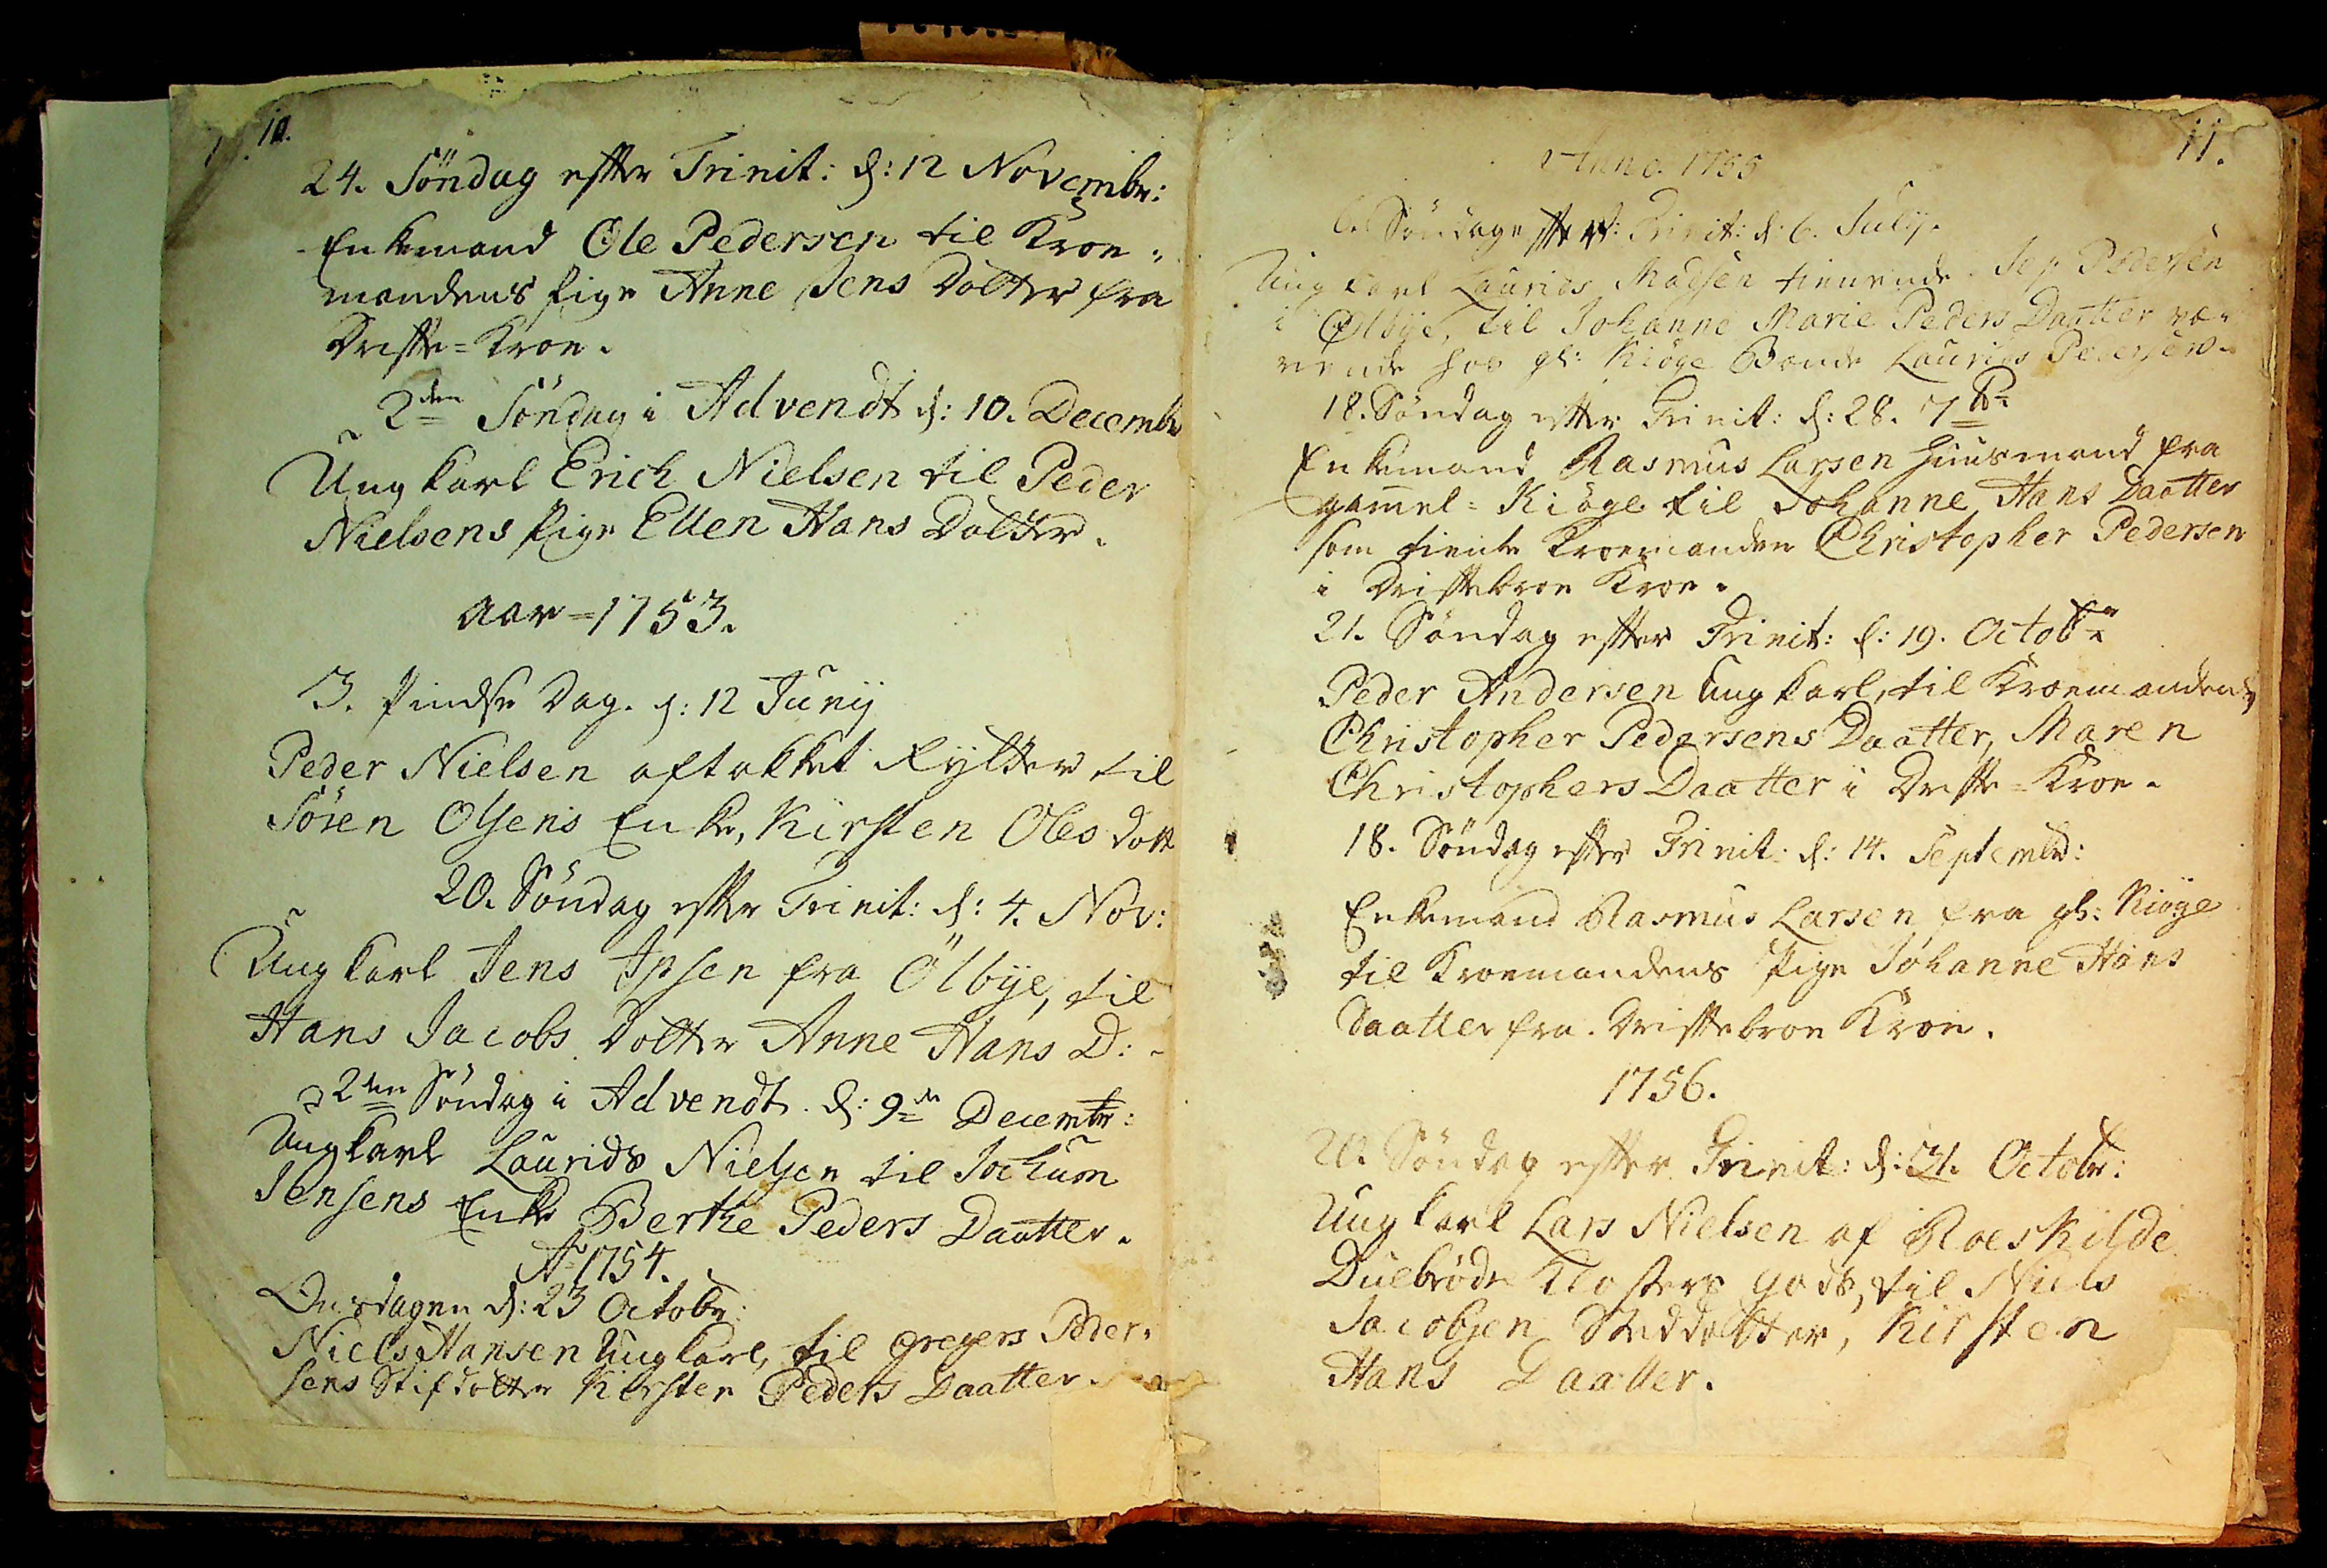10.
24. Søndag efter Trinit: d: 12 Novembr:
Enkemand Ole Pedersen til Kroe¬
mandens Pige Anne Jens Datter fra 
Drifte=Kroe.
2den Søndag i Advendt d: 10. Decembr
Ungkarl Erich Nielsen til Peder
Nielsens Pige Ellen Hans Datter.
Aar = 1753.
3. Pindse Dag. d: 12 Junij
Peder Nielsen aftakket Rytter til 
Søren Olsens Enke, Kirsten Olesdatt
20. Søndag efter Trinit: d: 4. Nov:
Ungkarl Jens Ipsen fra Ølbye, til
Hans Jacobs Datter Anne Hans D:
2den Søndag i Advendt. d: 9de Decembr:
Ungkarl Laurids Nielsen til Jochum
Jensens Enke Berthe Peders Daatter.
Ao 1754.
Onsdagen d: 23 Octobr:
Niels Hansen Ungkarl, til Gregers Peder¬
sens Stifdatter Kirsten Peders Daatter.
11.
Anno 1755
6. Søndag efter D: Trinit: d: 6. Julij.
Ungkarl Laurids Madsen tienende Jep Pedersen
i Ølbye, til Johanne Marie Peders Daatter væ¬
rende hos gl: Kiøge Bonde Laurids Pedersen.
18. Søndag efter Trinit: d: 28. 7br
Enkemand Rasmus Larsen Huusmand fra
Gammel = Kiøge til Johanne Hans Daatter
som tiente Kroemanden Christopher Pedersen
i Driftebroe Kroe.
21. Søndag efter Trinit: d: 19. Octobr.
Peder Andersen Ungkarl, til Kroemandens
Christopher Pedersens Daatter, Maren
Christophers Daatter i Drifte = Kroe.
18. Søndag efter Trinit: d: 14. Septembr:
Enkemand Rasmus Larsen fra gl: Kiøge
til Kroemandens Pige Johanne Hans
Daatter fra Driftebroe Kroe
1756.
20. Søndag efter Trinit: d: 31. Octobr:
Ungkarl Lars Nielsen af Roeskilde
Duebrødr Klosters Gods, til Niels
Jacobsen Steddatter, Kirsten
Hans Daatter. 
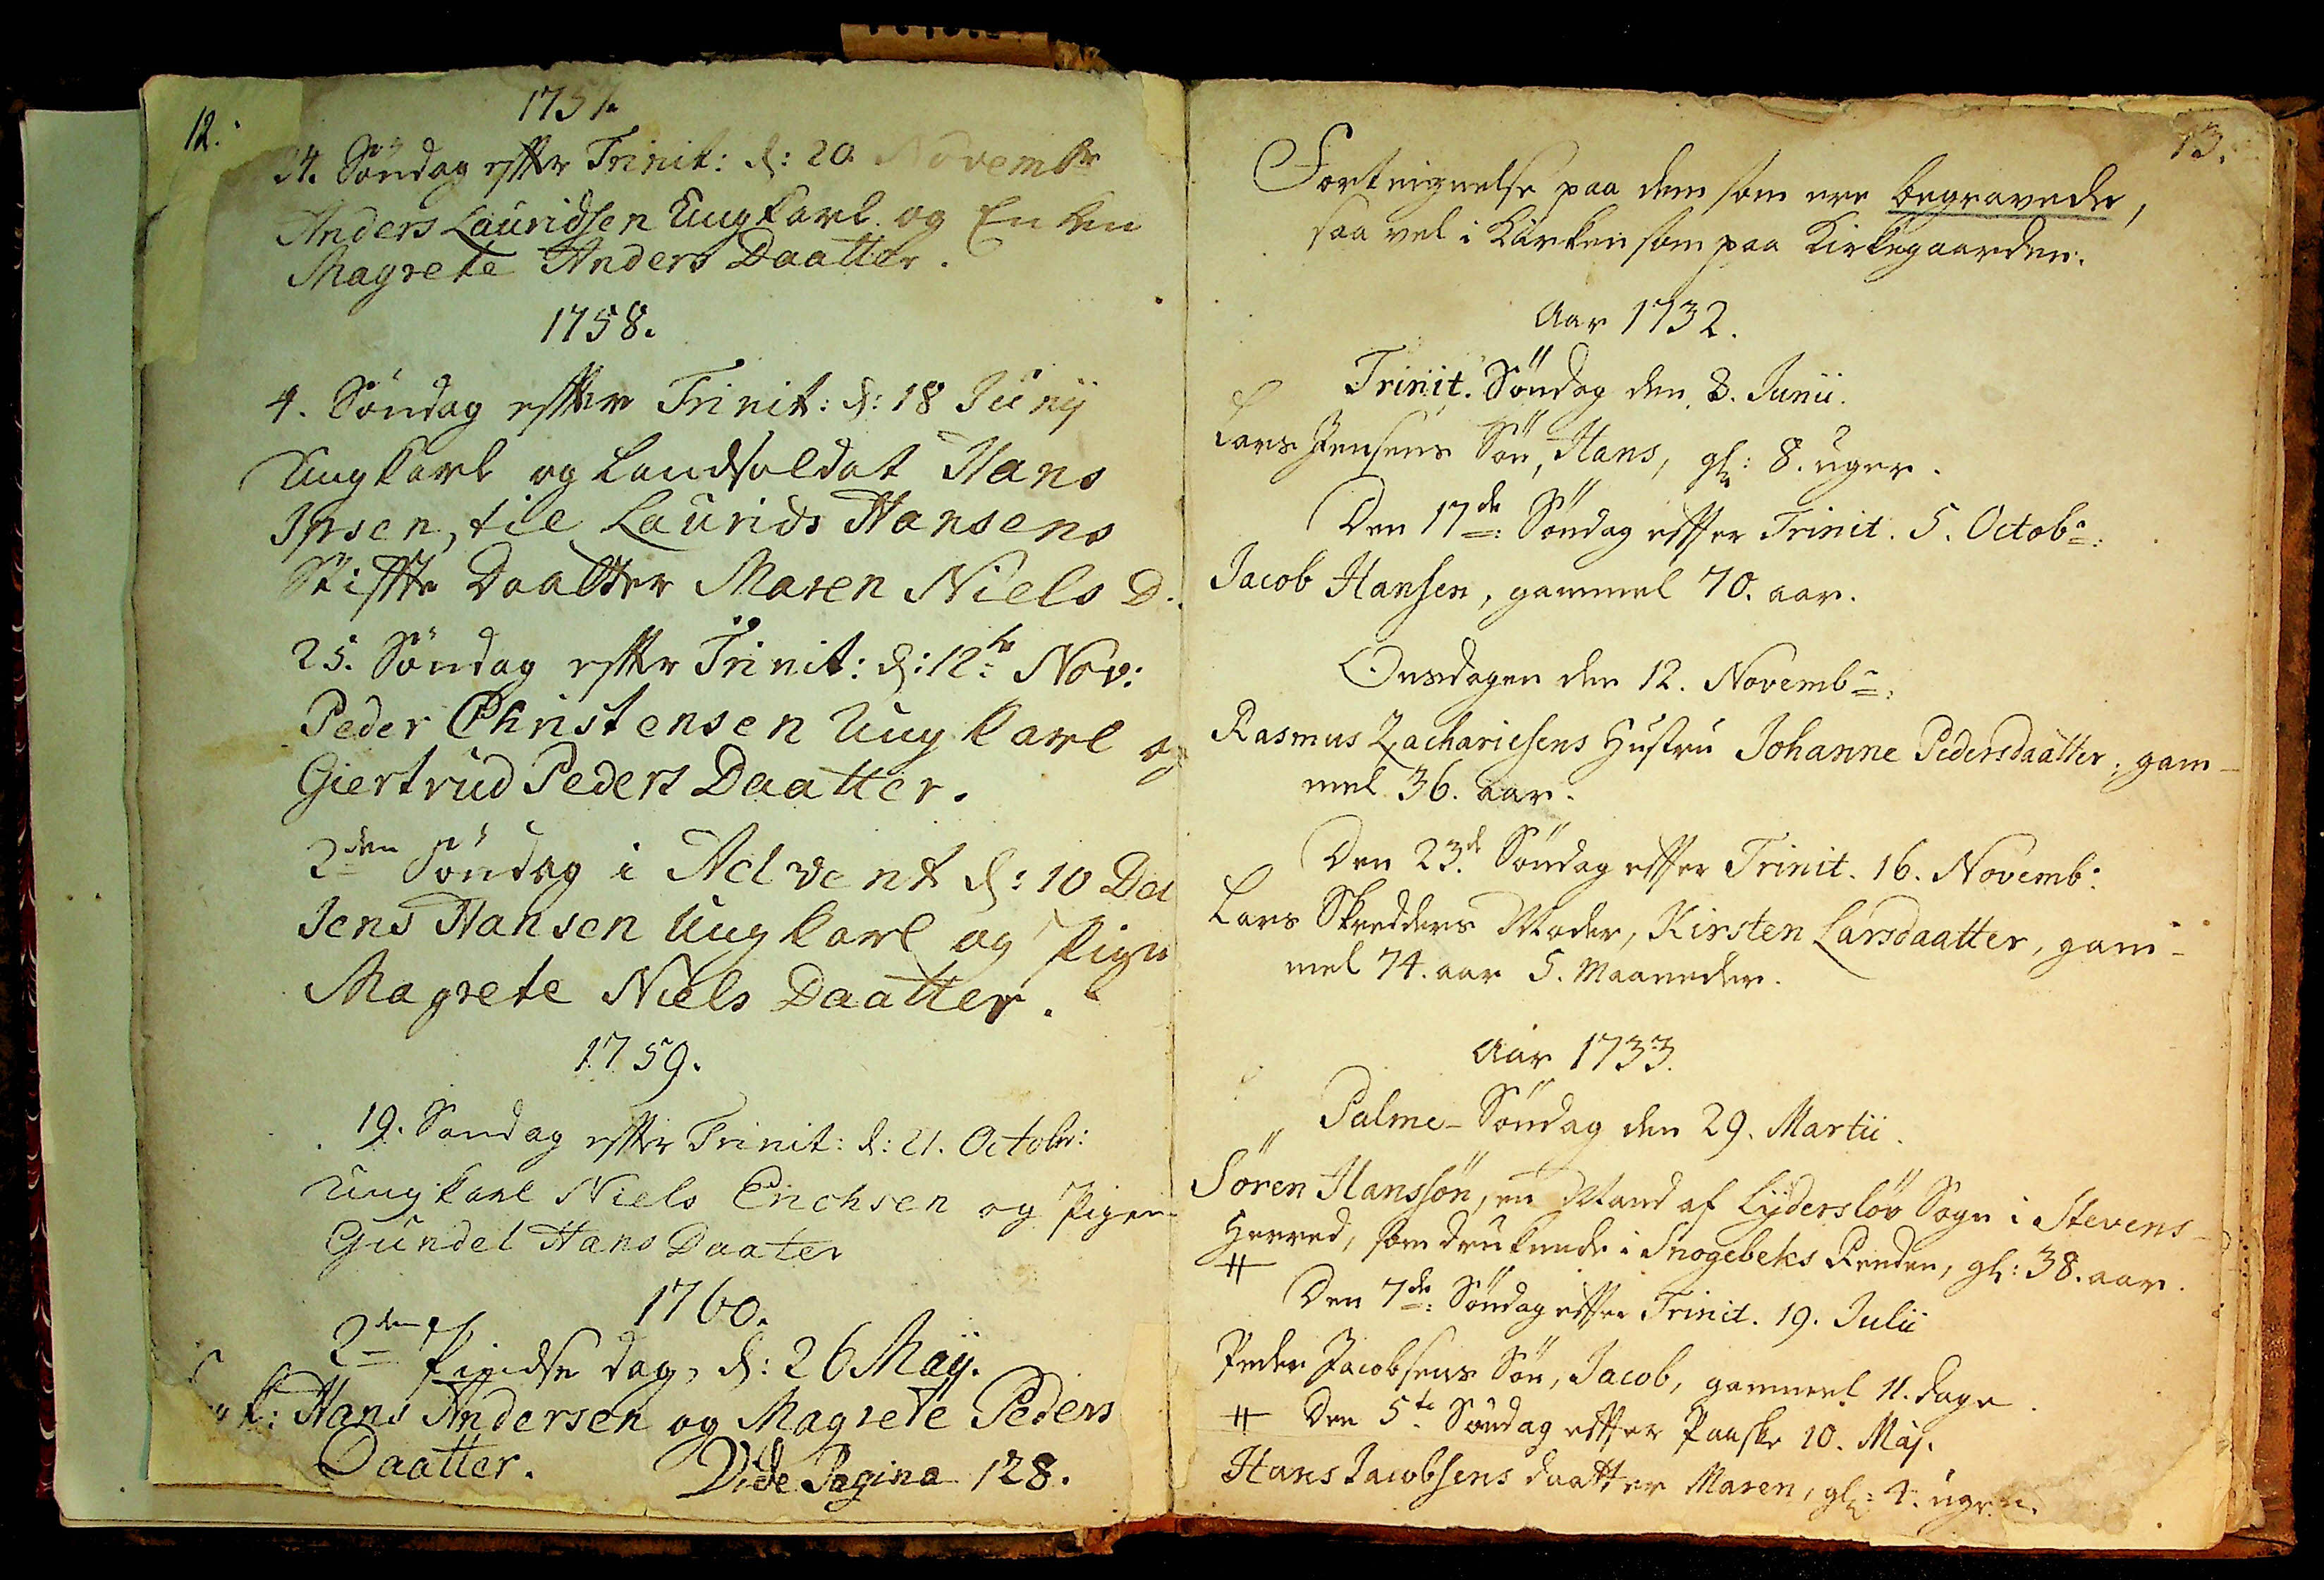12.
1757.
24. Søndag efter Trinit: d: 20. Novembr:
Anders Lauridsen Ungkarl og Enken
Magrete Anders Daatter.
1758.
4. Søndag efter Trinit: d: 18 Junij
Ungkarl og Landsoldat Hans
Ipsen, til Laurids Hansens
Stifte Daatter Maren Niels D.
25. Søndag efter Trinit: d: 12te Nov:
Peder Christensen Ung karl og
Giertrud Peders Daatter.
2den Søndag i Advent d: 10 Dec
Jens Hansen Ungkarl og Pige
Magrete Niels Daatter.
1759.
19. Søndag efter Trinit: d: 21. Octobr:
Ungkarl Niels Erichsen og Pigen
Gundel Hans Daatter.
1760.
2den Pindse Dag, d: 26 Maij.
Ungk: Hans Andersen og Magrete Peders
Daatter. 
Vide Pagina 128.
13.
Forteignelse paa dem som var begravede,
saa vel i Kirken som paa Kirkegaarden.
Aar 1732.
Trinit. Søndag den 8. Junii.
Lars Jensens Søn, Hans, gl: 8 uger.
Den 17de: Søndag efter Trinit. 5. Octobr:
Jacob Hansen, gammel 70 aar.
Onsdagen den 12. Novembr:
Rasmus Zachariesens Hustru Johanne Pedersdaatter. gam¬
mel 36. aar.
Den 23d Søndag efter Trinit. 16. Novemb:
Lars Skredders Moder, Kirsten Larsdaatter, gam¬
mel 74. aar 5. Maaneder.
Aar 1733.
Palme Søndag den 29. Martii.
Søren Hanssøn, en Mand af Lydersløv Sogn i Stevens
Herred, som druknede i Snogebeks Renden, gl: 38. aar.
Den 7de: Søndag efter Trinit. 19. Julii
Peder Jacobsens Søn, Jacob, gammel 11. dage.
Den 5te Søndag efter Paaske 10. Maj.
Hans Jacobsens daatter Maren, gl: 4. uger.